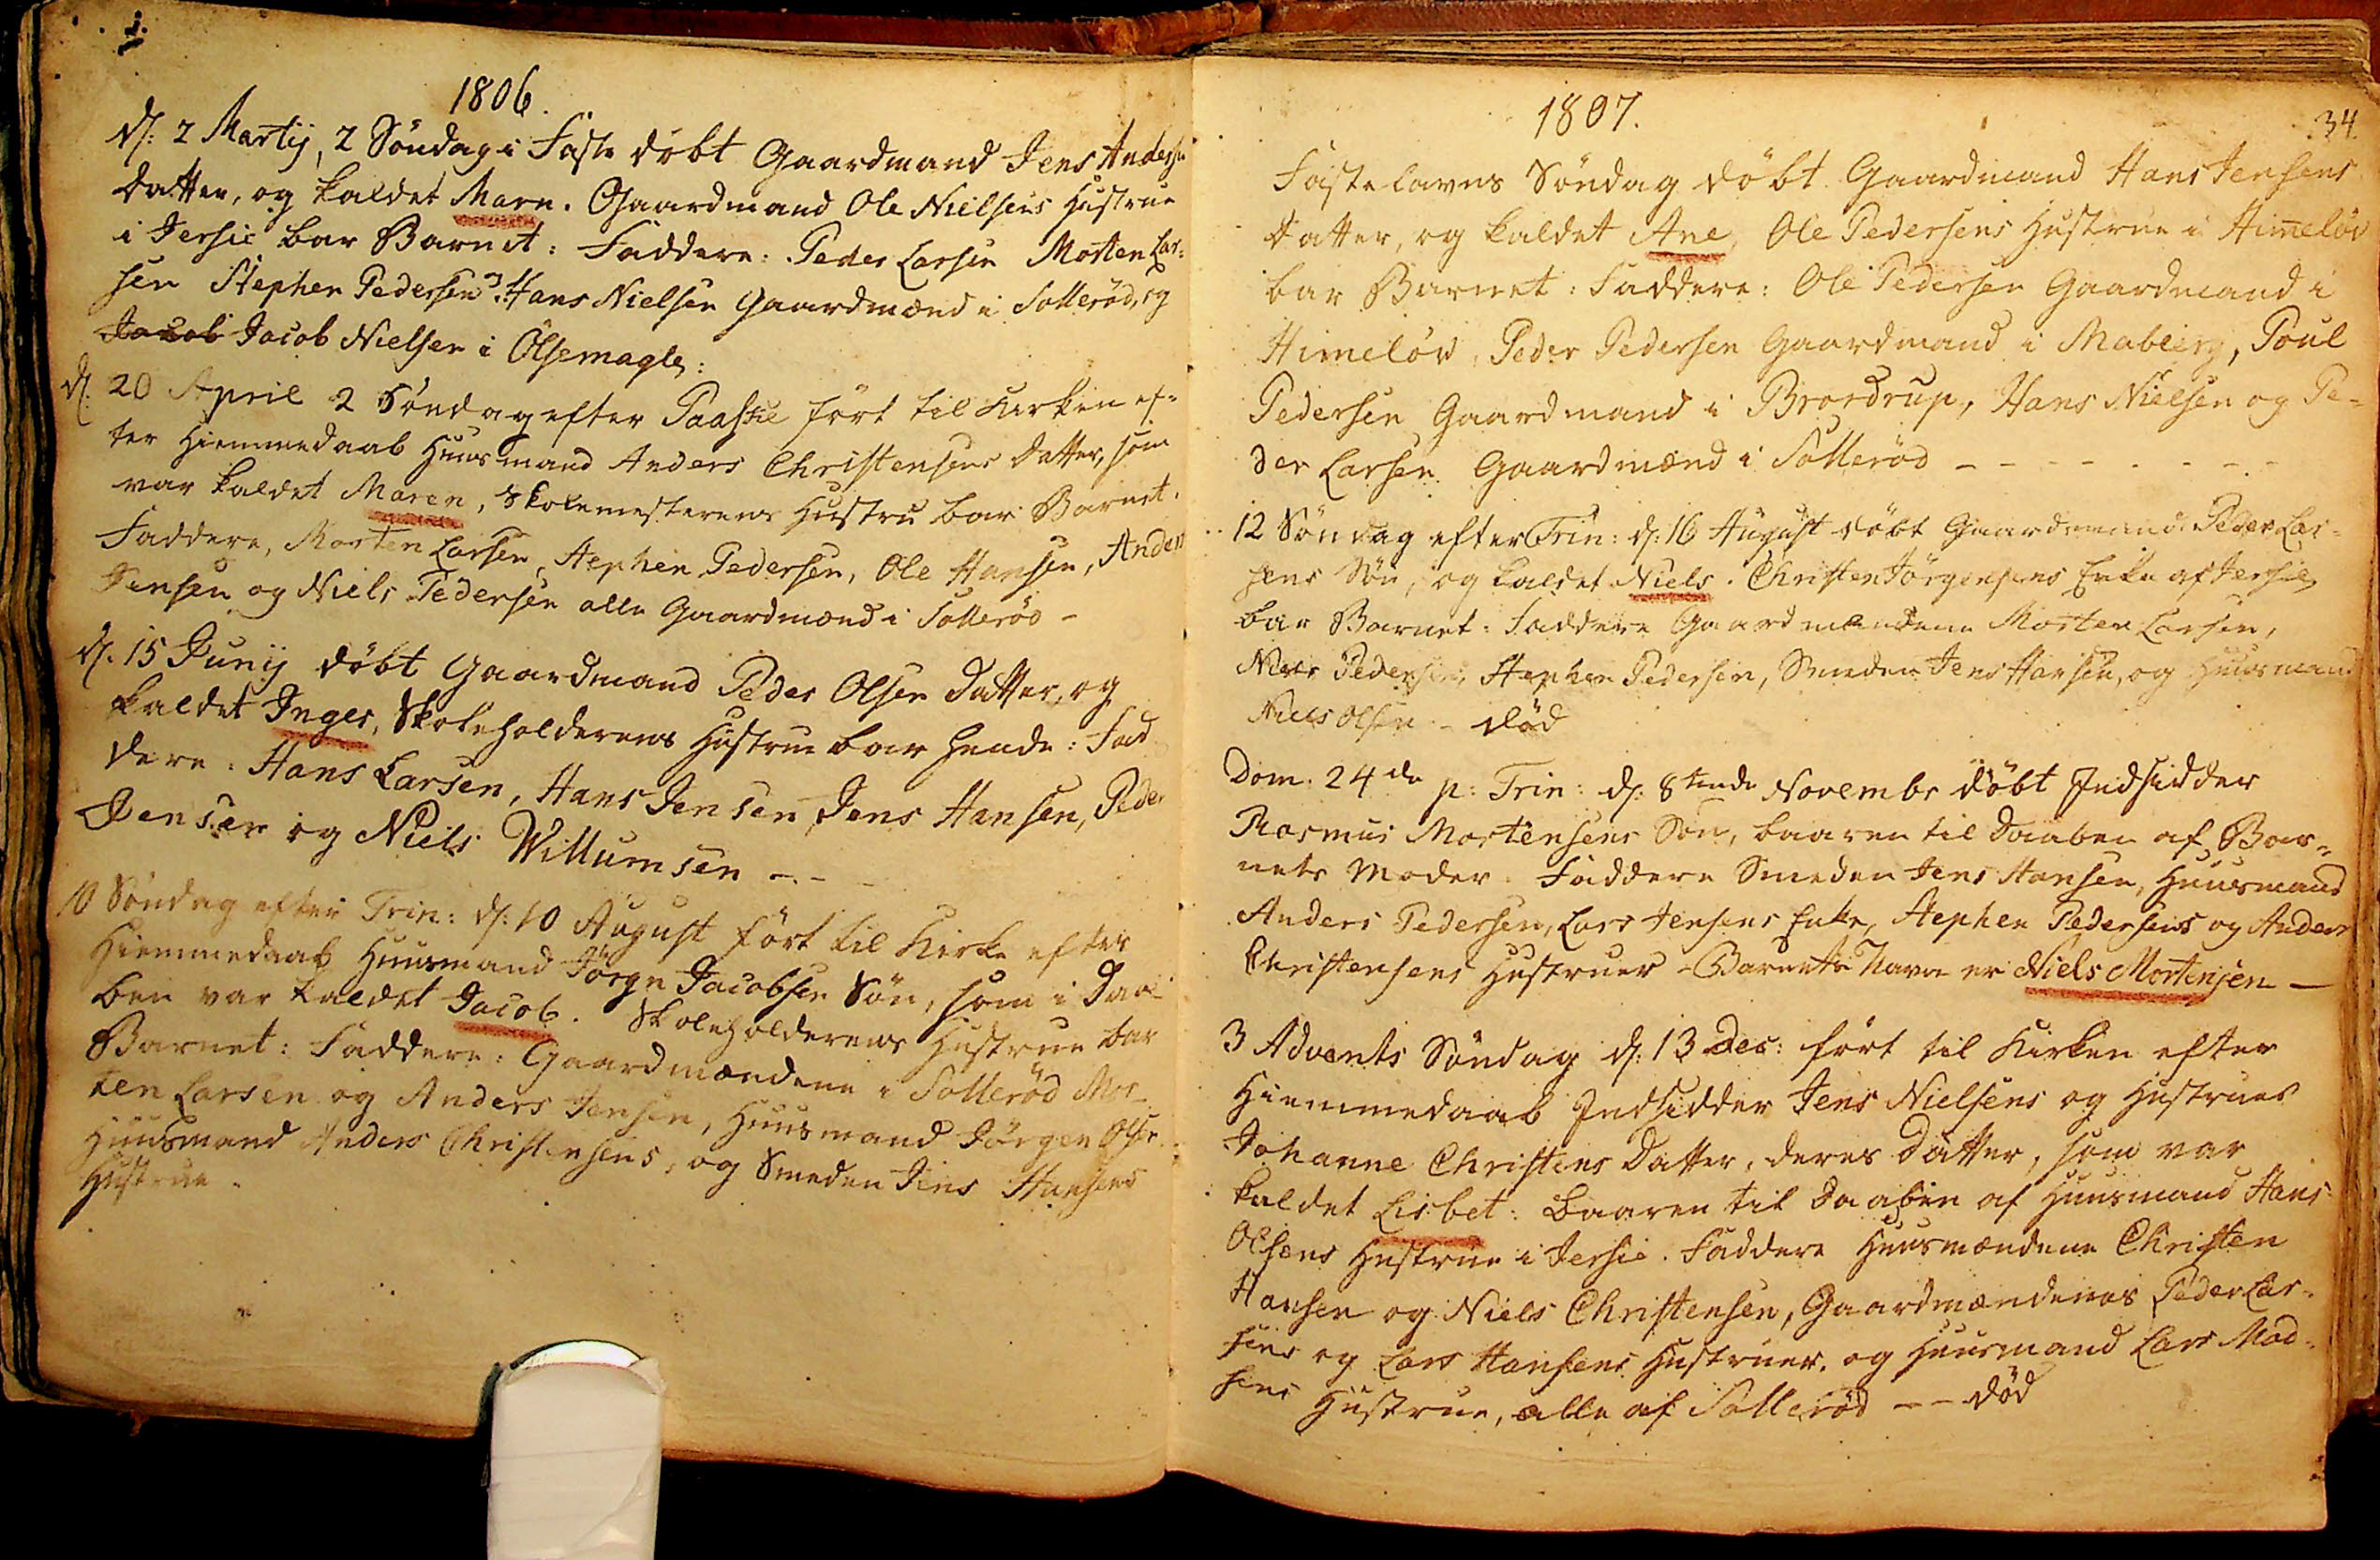1806.
d: 2 Martij, 2 Søndag i Faste døbt Gaardmand Jens Andersen
Datter, og kaldet Marn. Gaardmand Ole Nielsens Hustrue
i Jersie bar Barnet: Faddere. Peder Larsen Morten Lar¬
sen Stephen Pedersen og Hans Nielsen Gaardmand i Sollerød, og
Jacob Jacob Nielsen i Ølsemagle:
d: 20 April 2 Søndag efter Paaske ført til Kirken ef¬
ter Hiemmedaab Huusmand Anders Christensens Datter, som
var kaldet Marn, Skoleholderens Hustrue bar Barnet.
Faddere, Morten Larsen, Stephen Pedersen, Ole Hansen, Anders
Jensen og Niels Pedersen alle Gaardmænd i Sollerød
d: 15 Junij døbt Gaardmand Peder Olsen Datter, og
kaldet Inger, Skoleholderens Hustrue bar hende: Fad
dere: Hans Larsen, Hans Jensen, Jens Hansen, Peder
Jensen og Niels Willumsen
10 Søndag efter Trin: d: 10 August ført til Kirke efter
Hiemmedaab Huusmand Jørgn Jacobsen Søn, som i Daa
ben var kaldet Jacob. Skoleholderens Hustrue bar
Barnet: Faddere: Gaardmændene i Sollerød Mor
ten Larsen og Anders Jensen, Huusmand Jørgen Olsen
Huusmand Anders Christensens, og Smeden Jens Hansens
Hustruer.
34.
1807.
Fastelavns Søndag døbt Gaardmand Hans Jensens
Datter og kaldet Ane. Ole Pedersens Hustrue i Himmeløv
bar Barnet: Faddere: Ole Pedersen Gaardmand i
Himeløv. Peder Pedersen Gaardmand i Mabierg, Poul
Pedersen Gaardmand i Brordrup, Hans Nielsen og Pe¬
der Larsen Gaardmænd i Sollerød 
12 Søndag efter Trin: d: 16 August døbt Gaardmand Peder Lar¬
sens Søn og kaldet Niels. Christen Jørgensens Enke af Jersie
bar Barnet: Faddere Gaardmændene Morten Larsen,
Niels Pedersen, Stephen Pedersen, Smeden Jens Hansen, og Huusmand
Niels Olsen. død
Dom: 24de p: Trin: d: 8tende Novembr døbt Indsidder
Rasmus Mortensens Søn, baaren til Daaben af Bar¬
nets Moder: Faddere Smeden Jens Hansen, Huusmand
Anders Pedersen, Lars Jensens Enke, Stephen Pedersens og Anders
Christensens Hustruer - Barnets navn er Niels Mortensen
3 Advents Søndag d: 13 Dec: ført til Kirken efter
Hiemmedaab Indsidder Jens Nielsens og Hustrues
Johanne Christens Datter, deres Datter, som var
kaldet Lisbet: Baaren til Daaben af Huusmand Hans
Olsens Hustrue i Jersie. Faddere Huusmændene Christen
Hansen og Niels Christensen, Gaardmændenes Peder Lar¬
sens og Lars Hansens Hustruer, og Huusmand Lars Mad¬
sens Hustrue, alle af Sollerød
død
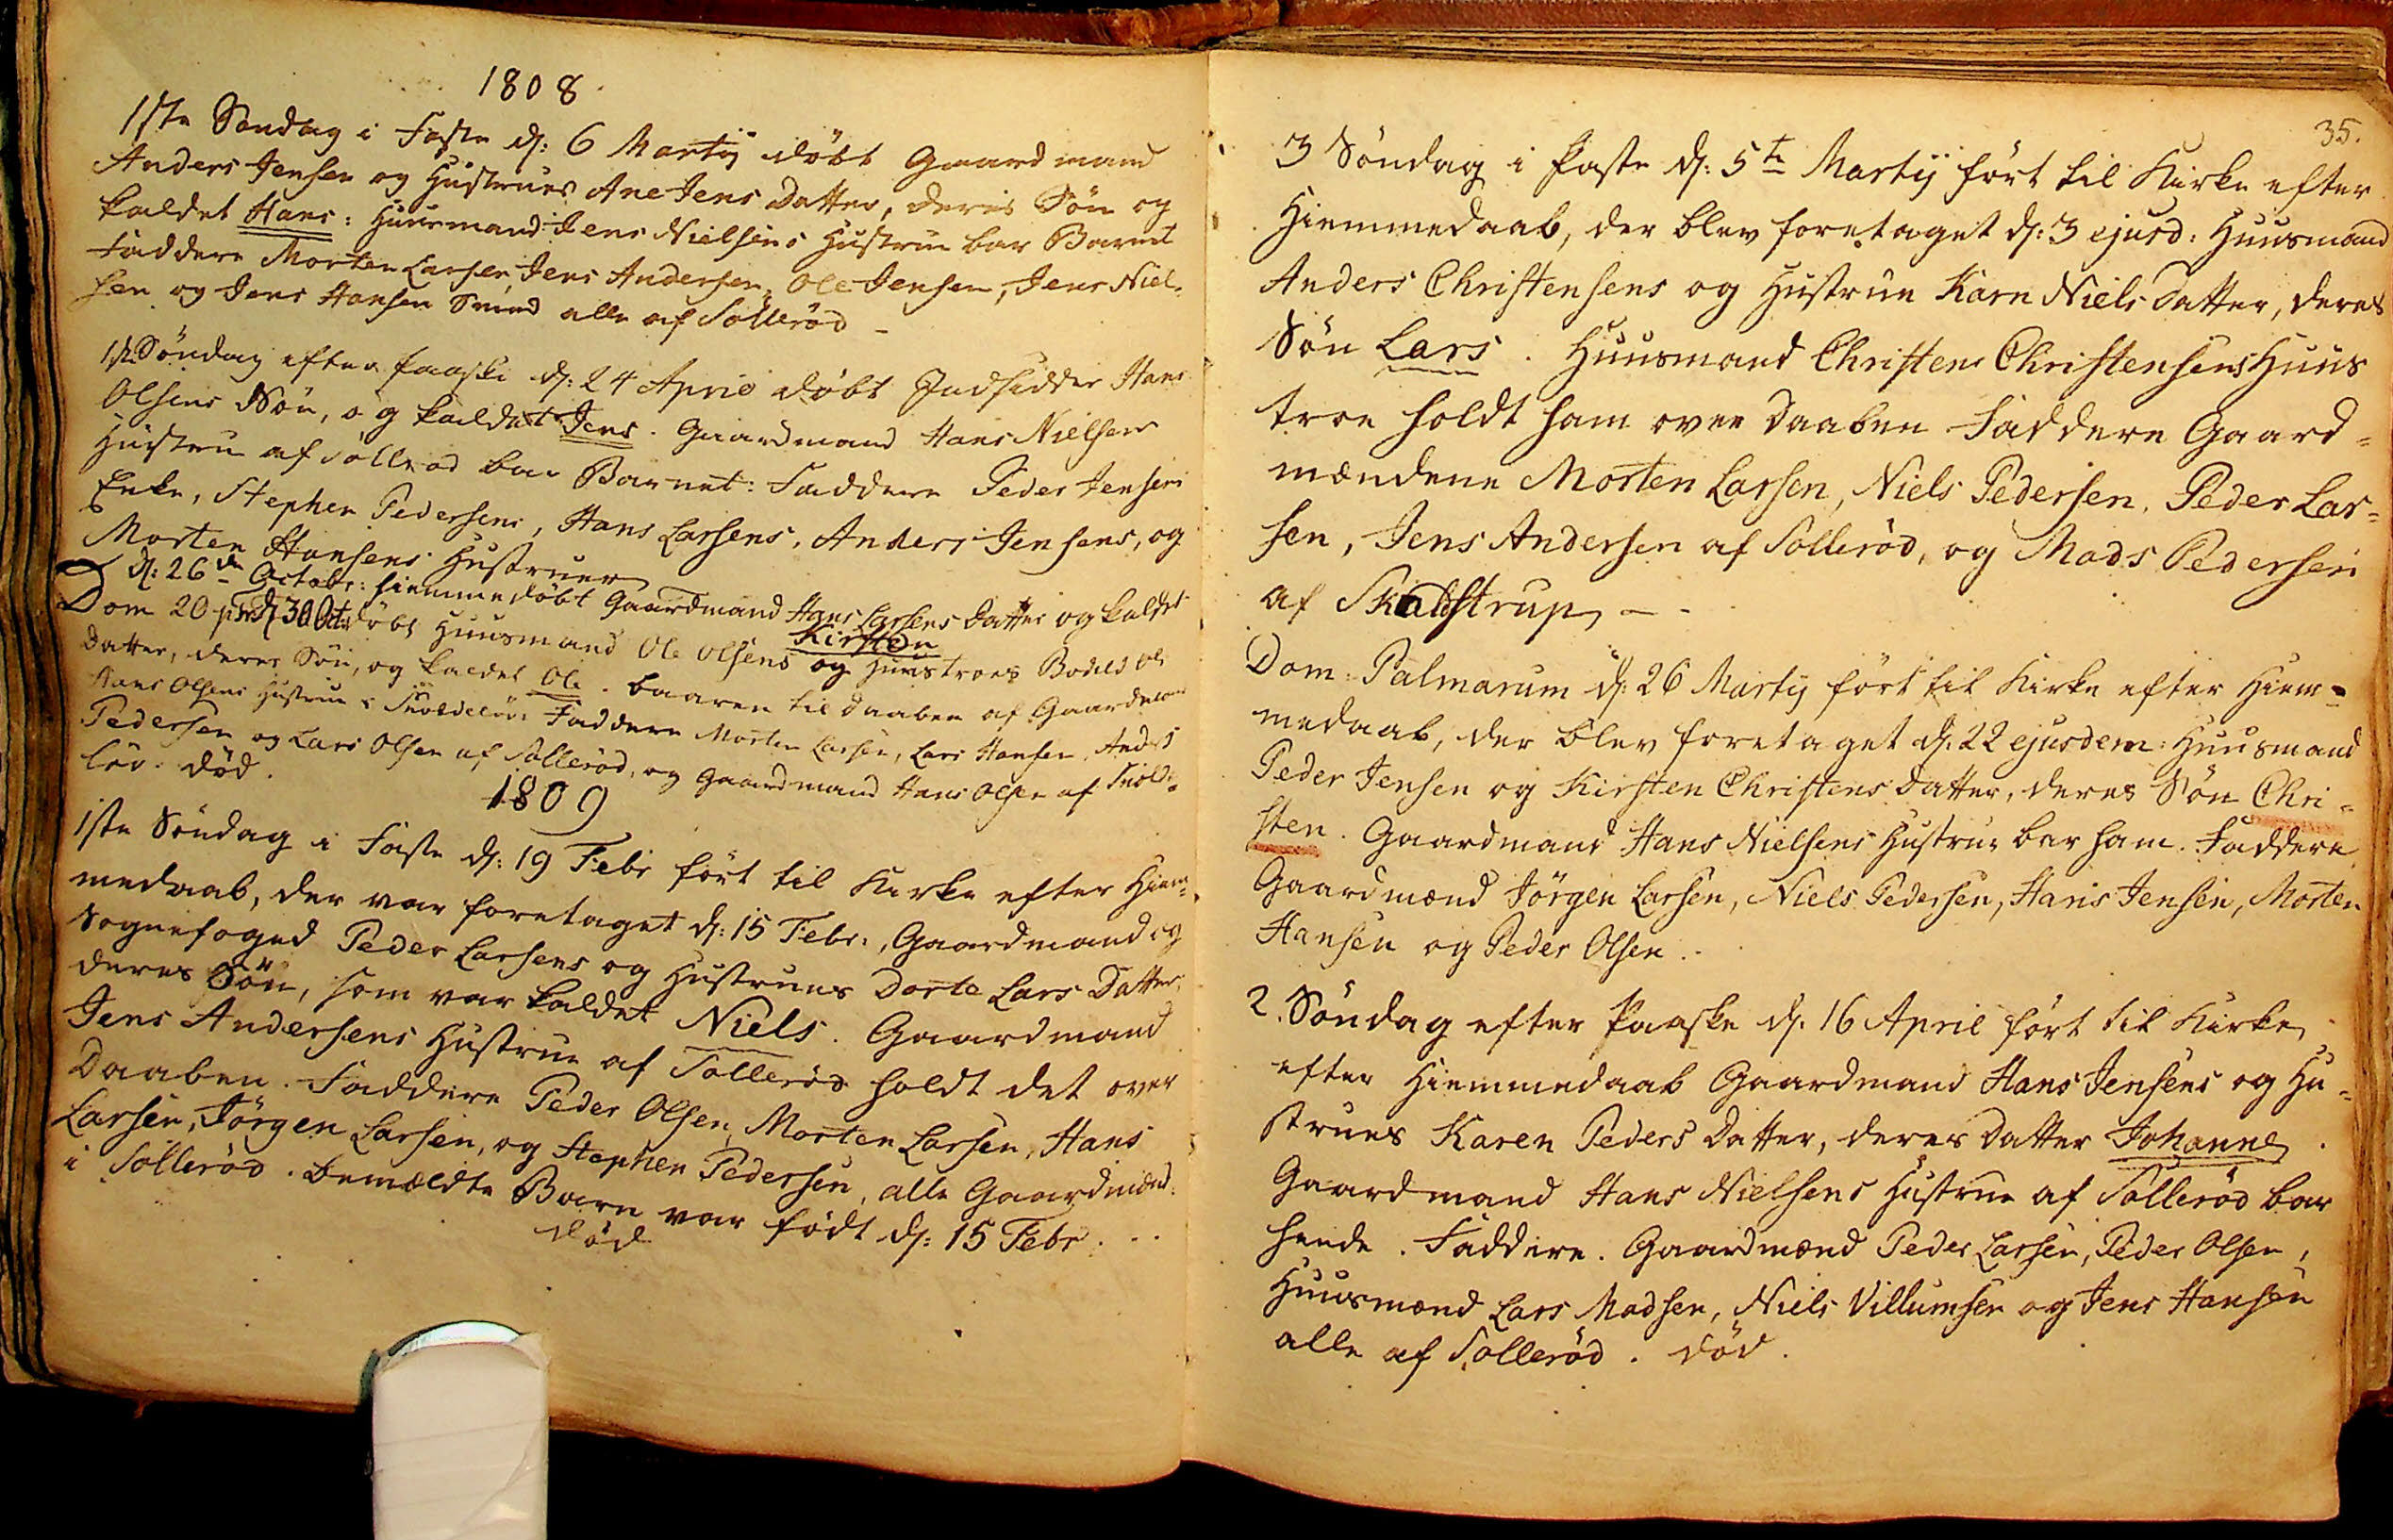1808
1ste Søndag i Faste d: 6 Martij døbt Gaardmand
Anders Jensen og Hustrues Ane Jens Datter, Deres Søn og
kaldet Hans: Huusmand Jens Nielsens Hustrue bar Barnet
Faddere Morten Larsen, Jens Andersen, Ole Jensen, Jens Niel¬
sen og Jens Hansen Smed alle af Sollerød
1ste Søndag efter Paaske d: 24 April døbt Indsidder Hans
Olsens Søn, og kaldet Jens. Gaardmand Hans Nielsens
Hustrue af Sollrød bar Barnet: Faddere Peder Jensens
Enke, Stephen Pedersens, Hans Larsens, Anders Jensens, og
Morten Hansens Hustruer
d: 26de Octobr: hiemmedøbt Gaardmand Hans Larsens Datter og kaldet
Kirsten
Dom 20 p Tr: d 30 Oct: døbt Huusmand Ole Olsens og Hustroes Bodild Ols
Datter, deres Søn, og kaldet Ole. baaren til Daaben af Gaardmand
Hans Olsens Hustrue i Snoldeløv: Faddere Morten Larsen, Lars Hansen Anders
Pedersen og Lars Olsen af Sollerød, og Gaardmand Hans Olsen af Snolde¬
løv. død
1809
1ste Søndag i Faste d: 19 Febr ført til Kirke efter Hiem¬
medaab, der var foretaget d: 15 Febr:, Gaardmand og
Sognefoged Peder Larsens og hustrues Dorte Lars Datter
deres Søn, som var kaldet Niels. Gaardmand
Jens Andersens Hustrue af Sollerød holdt det over
Daaben. Faddere Peder Olsen, Morten Larsen, Hans
Larsen, Jørgen Larsen, og Stephen Pedersen, alle Gaardmænd
i Sollerød. Bemældte Barn var født d: 15 Febr.
død
35.
3 Søndag i Faste d: 5te Martij ført til Kirke efter
Hiemmedaab, der var foretaget d: 3 ejusd: Huusmand
Anders Christensens og Hustrue Karn Niels Datter, deres
Søn Lars. Huusmand Christen Christensens Huus
troe holdt ham over Daaben. Faddere Gaard¬
mændene Morten Larsen, Niels Pedersen, Peder Lar¬
sen, Jens Andersen af Sollerød og Mads Pedersen
af Skaldstrup
Dom: Palmarum d: 26 Martij ført til Kirke efter Hiem¬
medaab, der blev foretaget d: 22 Ejusdem: Huusmand
Peder Jensen og Kirsten Christens Datter, deres Søn Chri¬
sten. Gaardmand Hans Nielsens Hustrue bar ham. Faddere
Gaardmand Jørgen Larsen, Niels Pedersen, Hans Jensen, Morten
Hansen og Peder Olsen.
2. Søndag efter Paaske d: 16 April ført til Kirke
efter Hiemmedaab Gaardmand Hans Jensens og Hu¬
strues Karen Peders Datter, deres Datter Johanne.
Gaardmand Hans Nielsens Hustrue af Sollerød bar
hende. Faddere. Gaardmænd Peder Larsen, Peder Olsen,
Huusmænd Lars Madsen, Niels Villumsen og Jens Hansen
alle af Sollerød. død